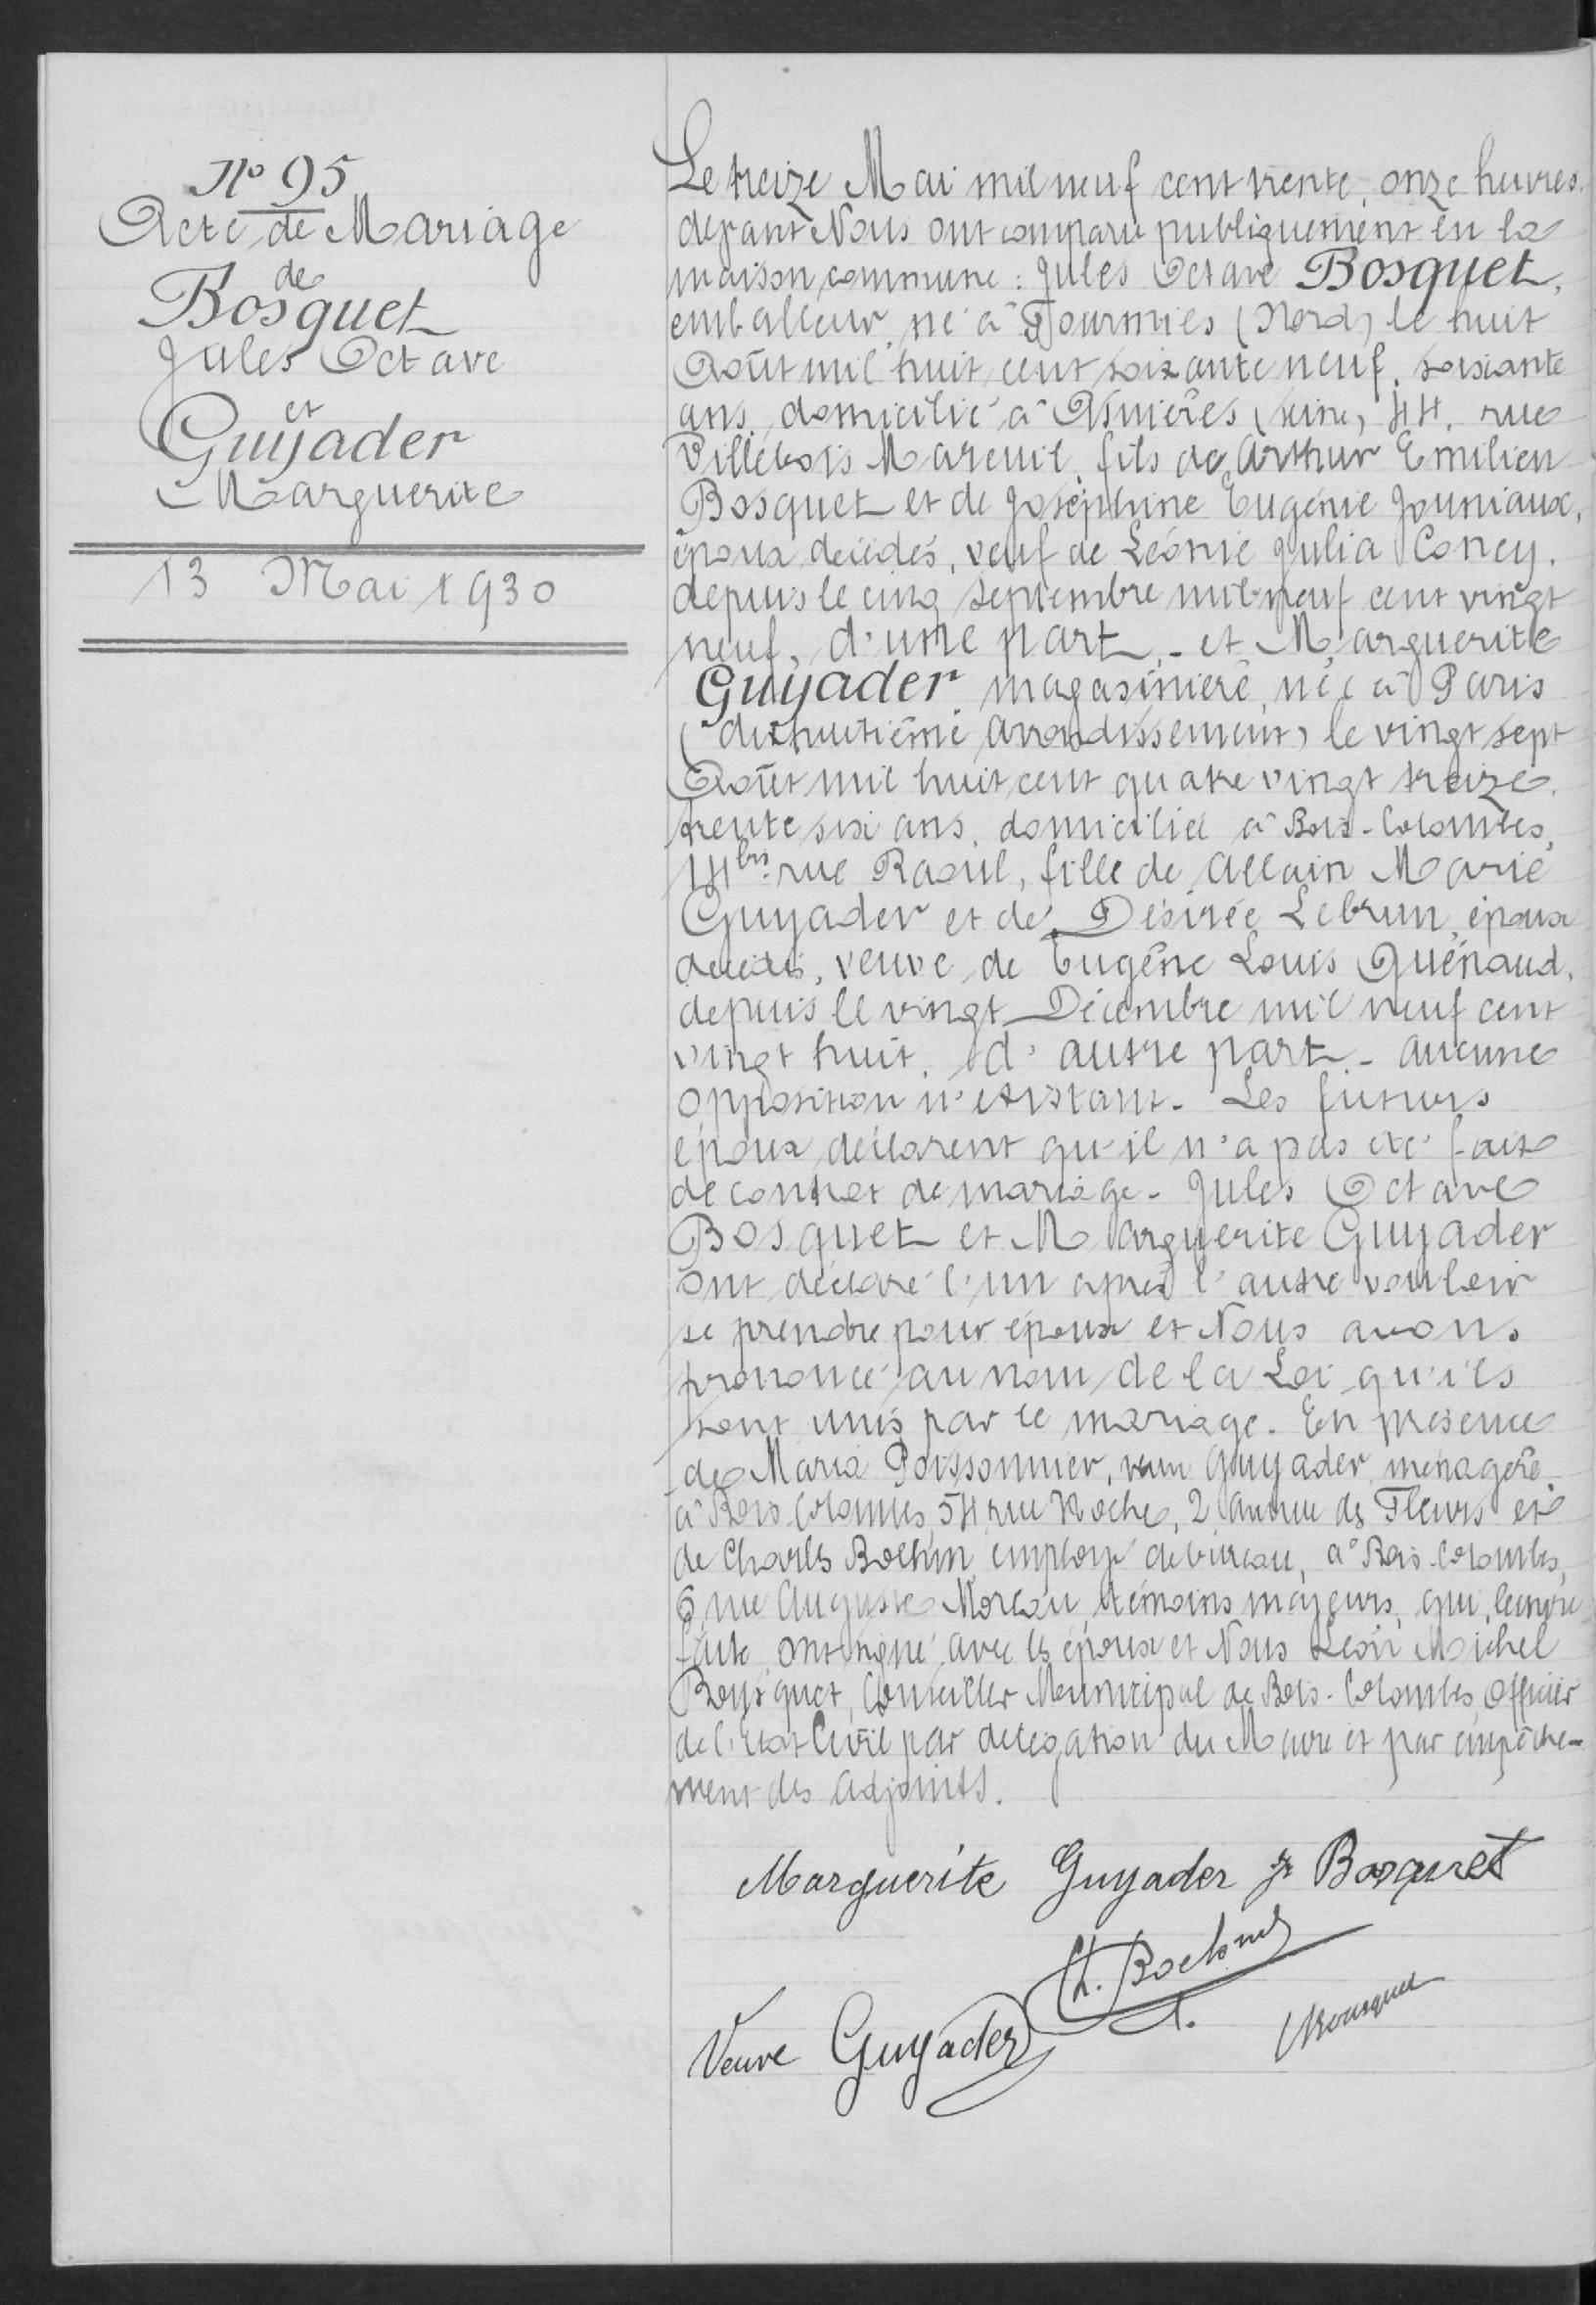N°95
Acte de Mariage
de
Bosquet
Jules Octave
et
Guijader
Marguerité
13 Mai 1930
Le treize Mai mil neuf cent trente, onze heures
devant Nous ont comparu publiquement en la
maison commune: Jules Octave Bosquet
emballeur, né à Gournes (Nord) le huit
août mil huit cent soixante neuf, soixante
ans, domicilié à Ainières, (Seine) 44, rue
Villebois-Mareuve, fils de Arthur Emilien
Bosquet et de Joséphine Eugénie Gouniaux,
époux décédés, veuf de Léonie Gulia Coney
depuis me cinq décembre mil neuf cent vingt
neuf, d'une part et Marguerite
Guyader, magasinière, née à Paris
(dixhuitième arrondissement) le vingt sept
août mil huit cent quatre vingt treize
trente six ans, domicilié à Bas-Colombe
14 bis rue Raoul, fille de Allain Marie
Guyader et de Désirée Lebrun, époux
décédé, veuve de Eugène Louis Guénaud,
depuis le vingt Décembre mil neuf cent
vingt huit, d'autre part .-aucune
opposition n'existant. Les futurs
époux déclarent qu'il n'a pas été fait
de contrat de mariage. Jules Octave
Bosquet et Marguerite Guyader
ont déclaré l'un après l'autre vouloir
se prendre pour époux et Nous avons
prononcé au nom de la Loi qu'il
sont unis par le mariage. En présence
de Maria Poissonnier, veuve Guyader ménagère
à Bois Colombes 54 rie Roche, 2 avenue des Fleurs ete
de Charles Bothin, employé de burreau, a° Bois-Colombes,
6 rue Auguste Morcaux témoins majeurs, qui, lecture
faite ont signé avec les époux et Nous Léon Michel
Busquet, Conseiller Municipal de Bois-Coolombes Officier
de l'Etat Civil par délégation du Maire et par empêche-
ment des adjoints.
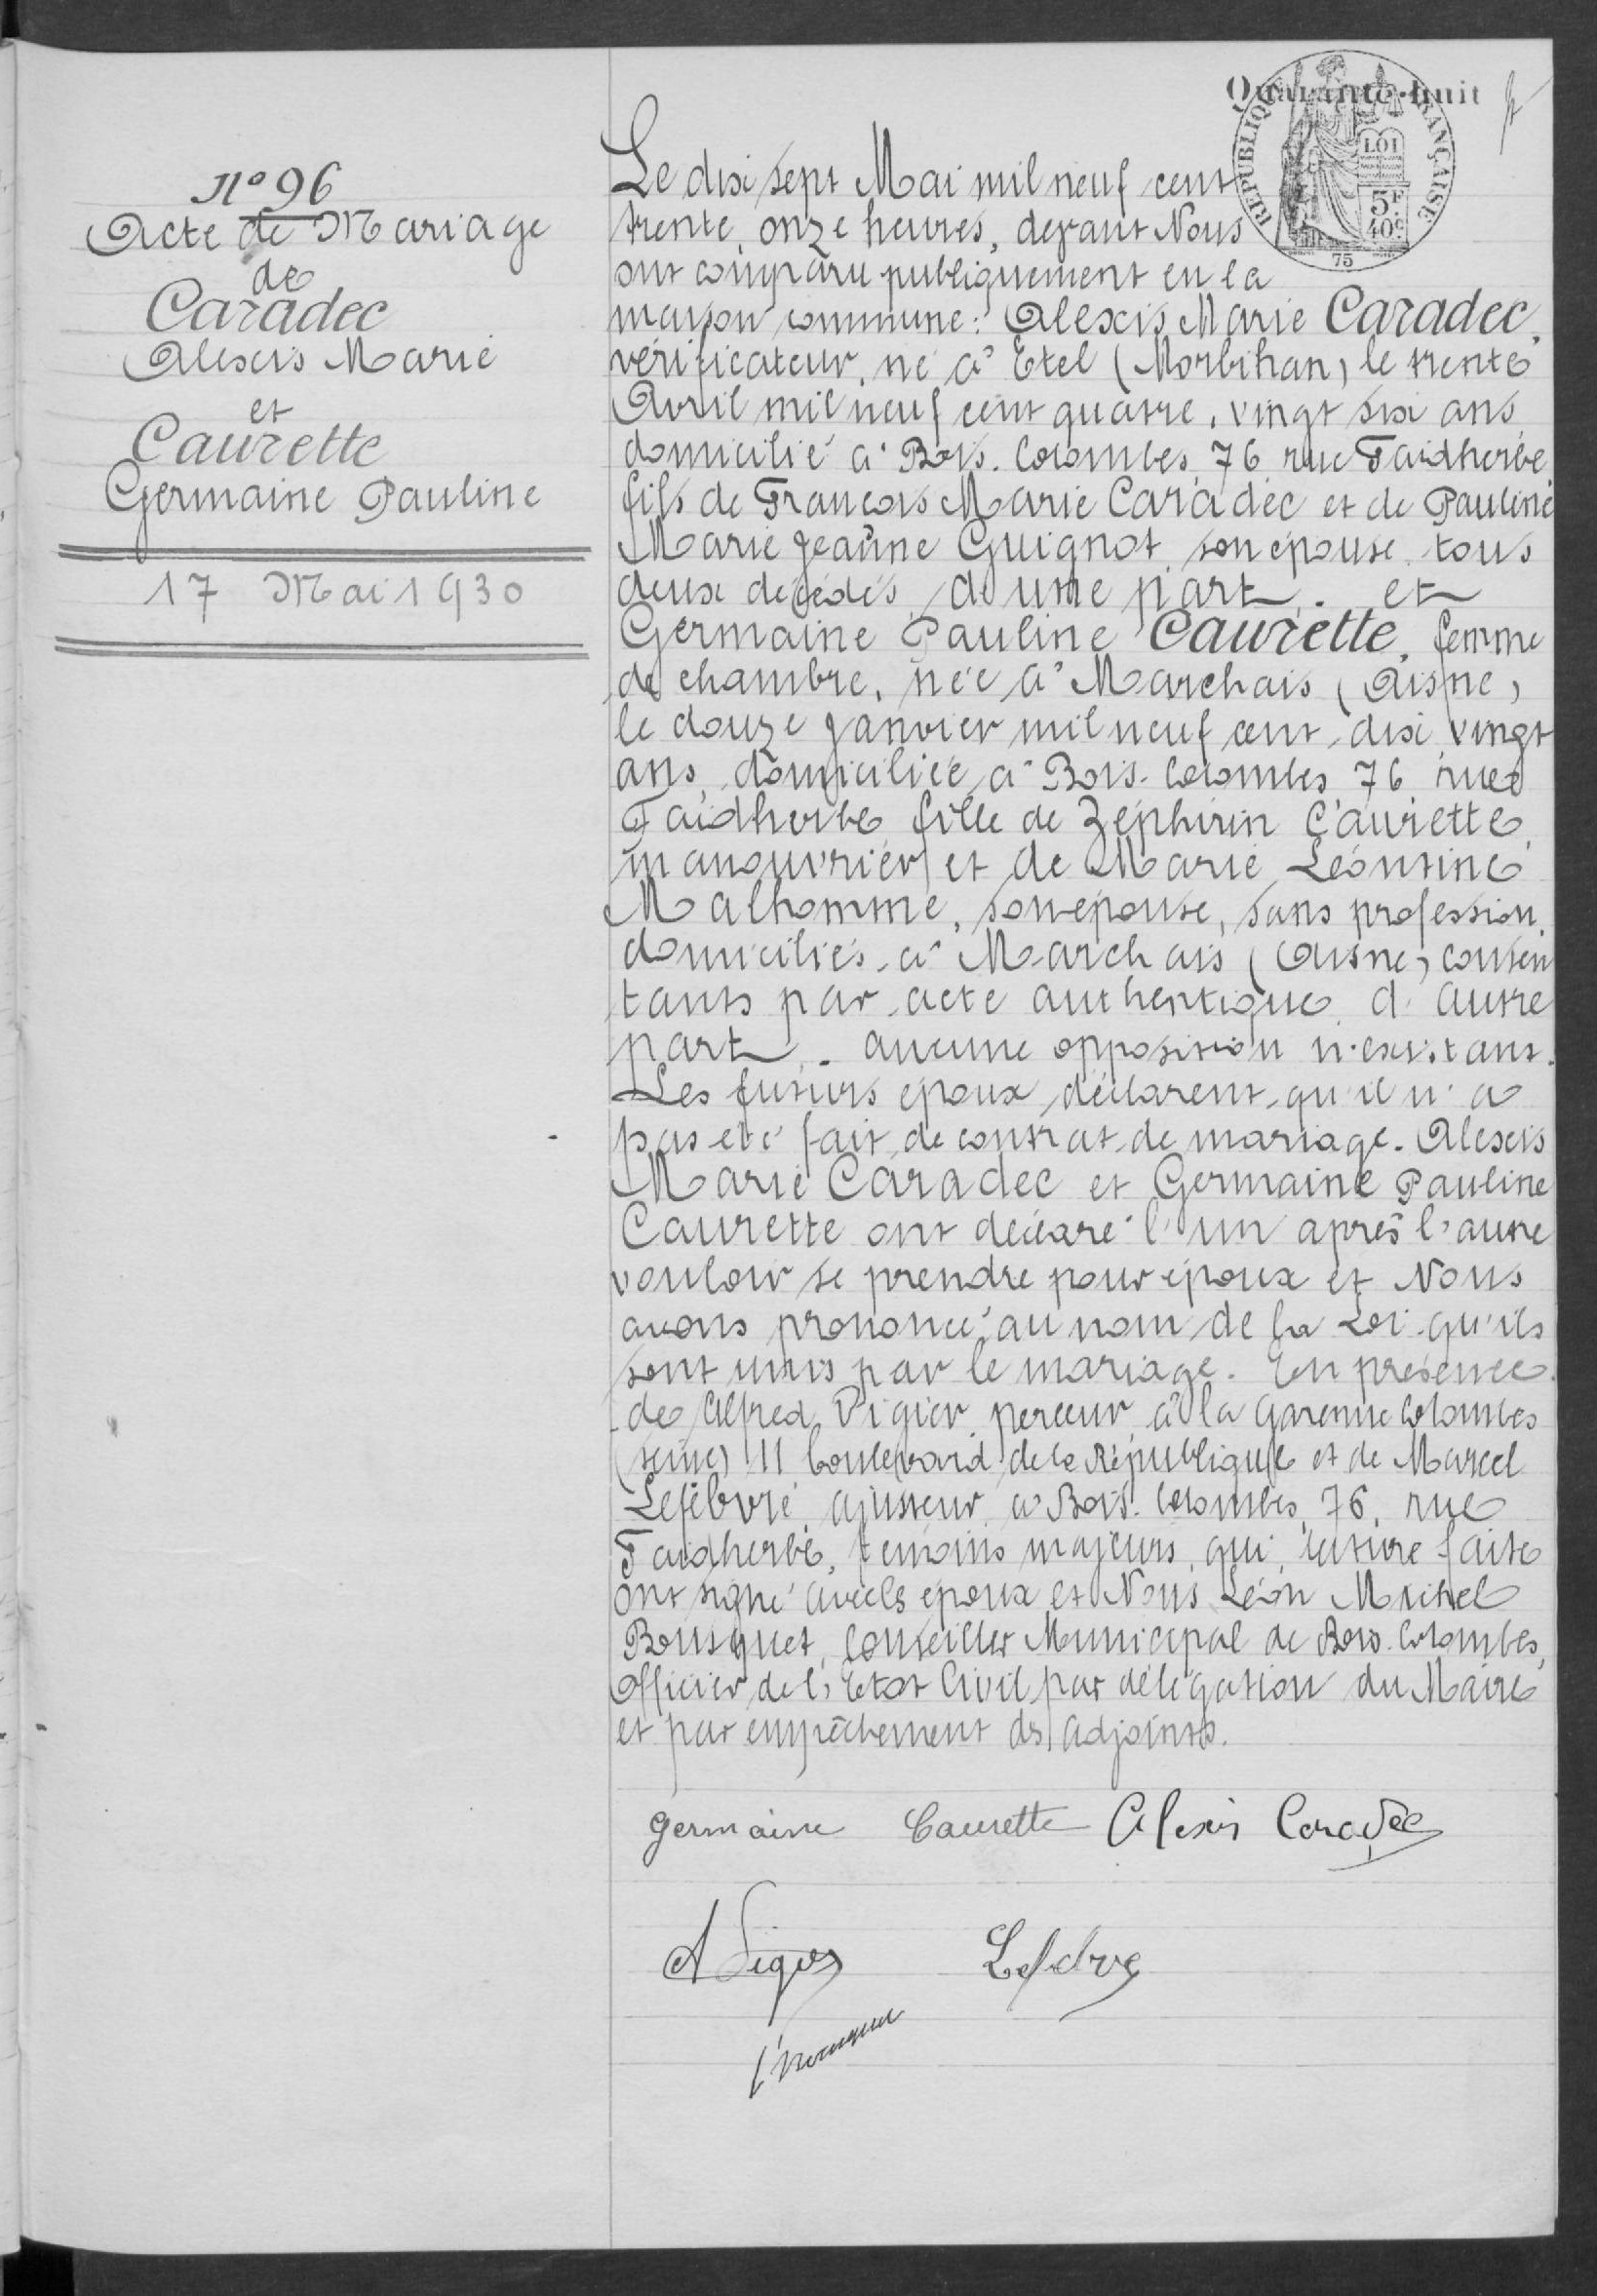N°96
Acte de Mariage
de
Caradec
Alexis Marie
et
Caurette
Germaine Pauline
17 Mai 1930
Le dix sept Mai mil neuf cent
trente, onze heures, devant Nous
ont comparu publiquement en la
maison commune: Alexis Marie Caradec
vérificateur, né à Etel (Morbihan) le trente
Avril mil neuf cent quatre, vingt six ans
domicilié à Bois-Colombes, 76, rue Faidhebre
fils de Françoise Marie Caradée et de Pauline
Marie Jeanne Guignot son épouse, tous
deux décédés, d'une part. et
germaine Pauline Caurette, femme
de chambre, née à Marehais (Aisne)
le douze janvier mil neuf cent dix, vingt
ans, domiciliée à Bois-Colombes 76 rue
Faidherbe fille de Zéphirin Caurette
manouvrier et de Marie Léonine
Malhomme, son épouse, sans profession
domiciliés à Marchais (Aisne) consen
tants par acte authentique d'autre
part. aucune opposition n'existant
Les futurs époux, déclarent, qu'il n'a
pas été fait de contrat de mariage. Alexis
Marie Caradec et Germaine Pauline
Caurette ont déclaré l'un après l'autre
vouloir se prendre pour époux et Nous
avons prononcé au nom de la Loi qu'ils
sont unis par le mariage. En présence
de Alfred Vigier, perceur à la Garenne Colombes
(Seine) 11 boulevard de la République et de Marcel
Lefebvre, agresseur à Bois Colombes, 76, rue
Faidherbe, témoins majeurs, qui, lecture faite
ont signé avec les époux et Nous Léon Michel
Bouquet, Conseiller Municipal de Bois Colombes
Officier de l'Etat Civil par délégation du Maire
et par empêchement des adjoints